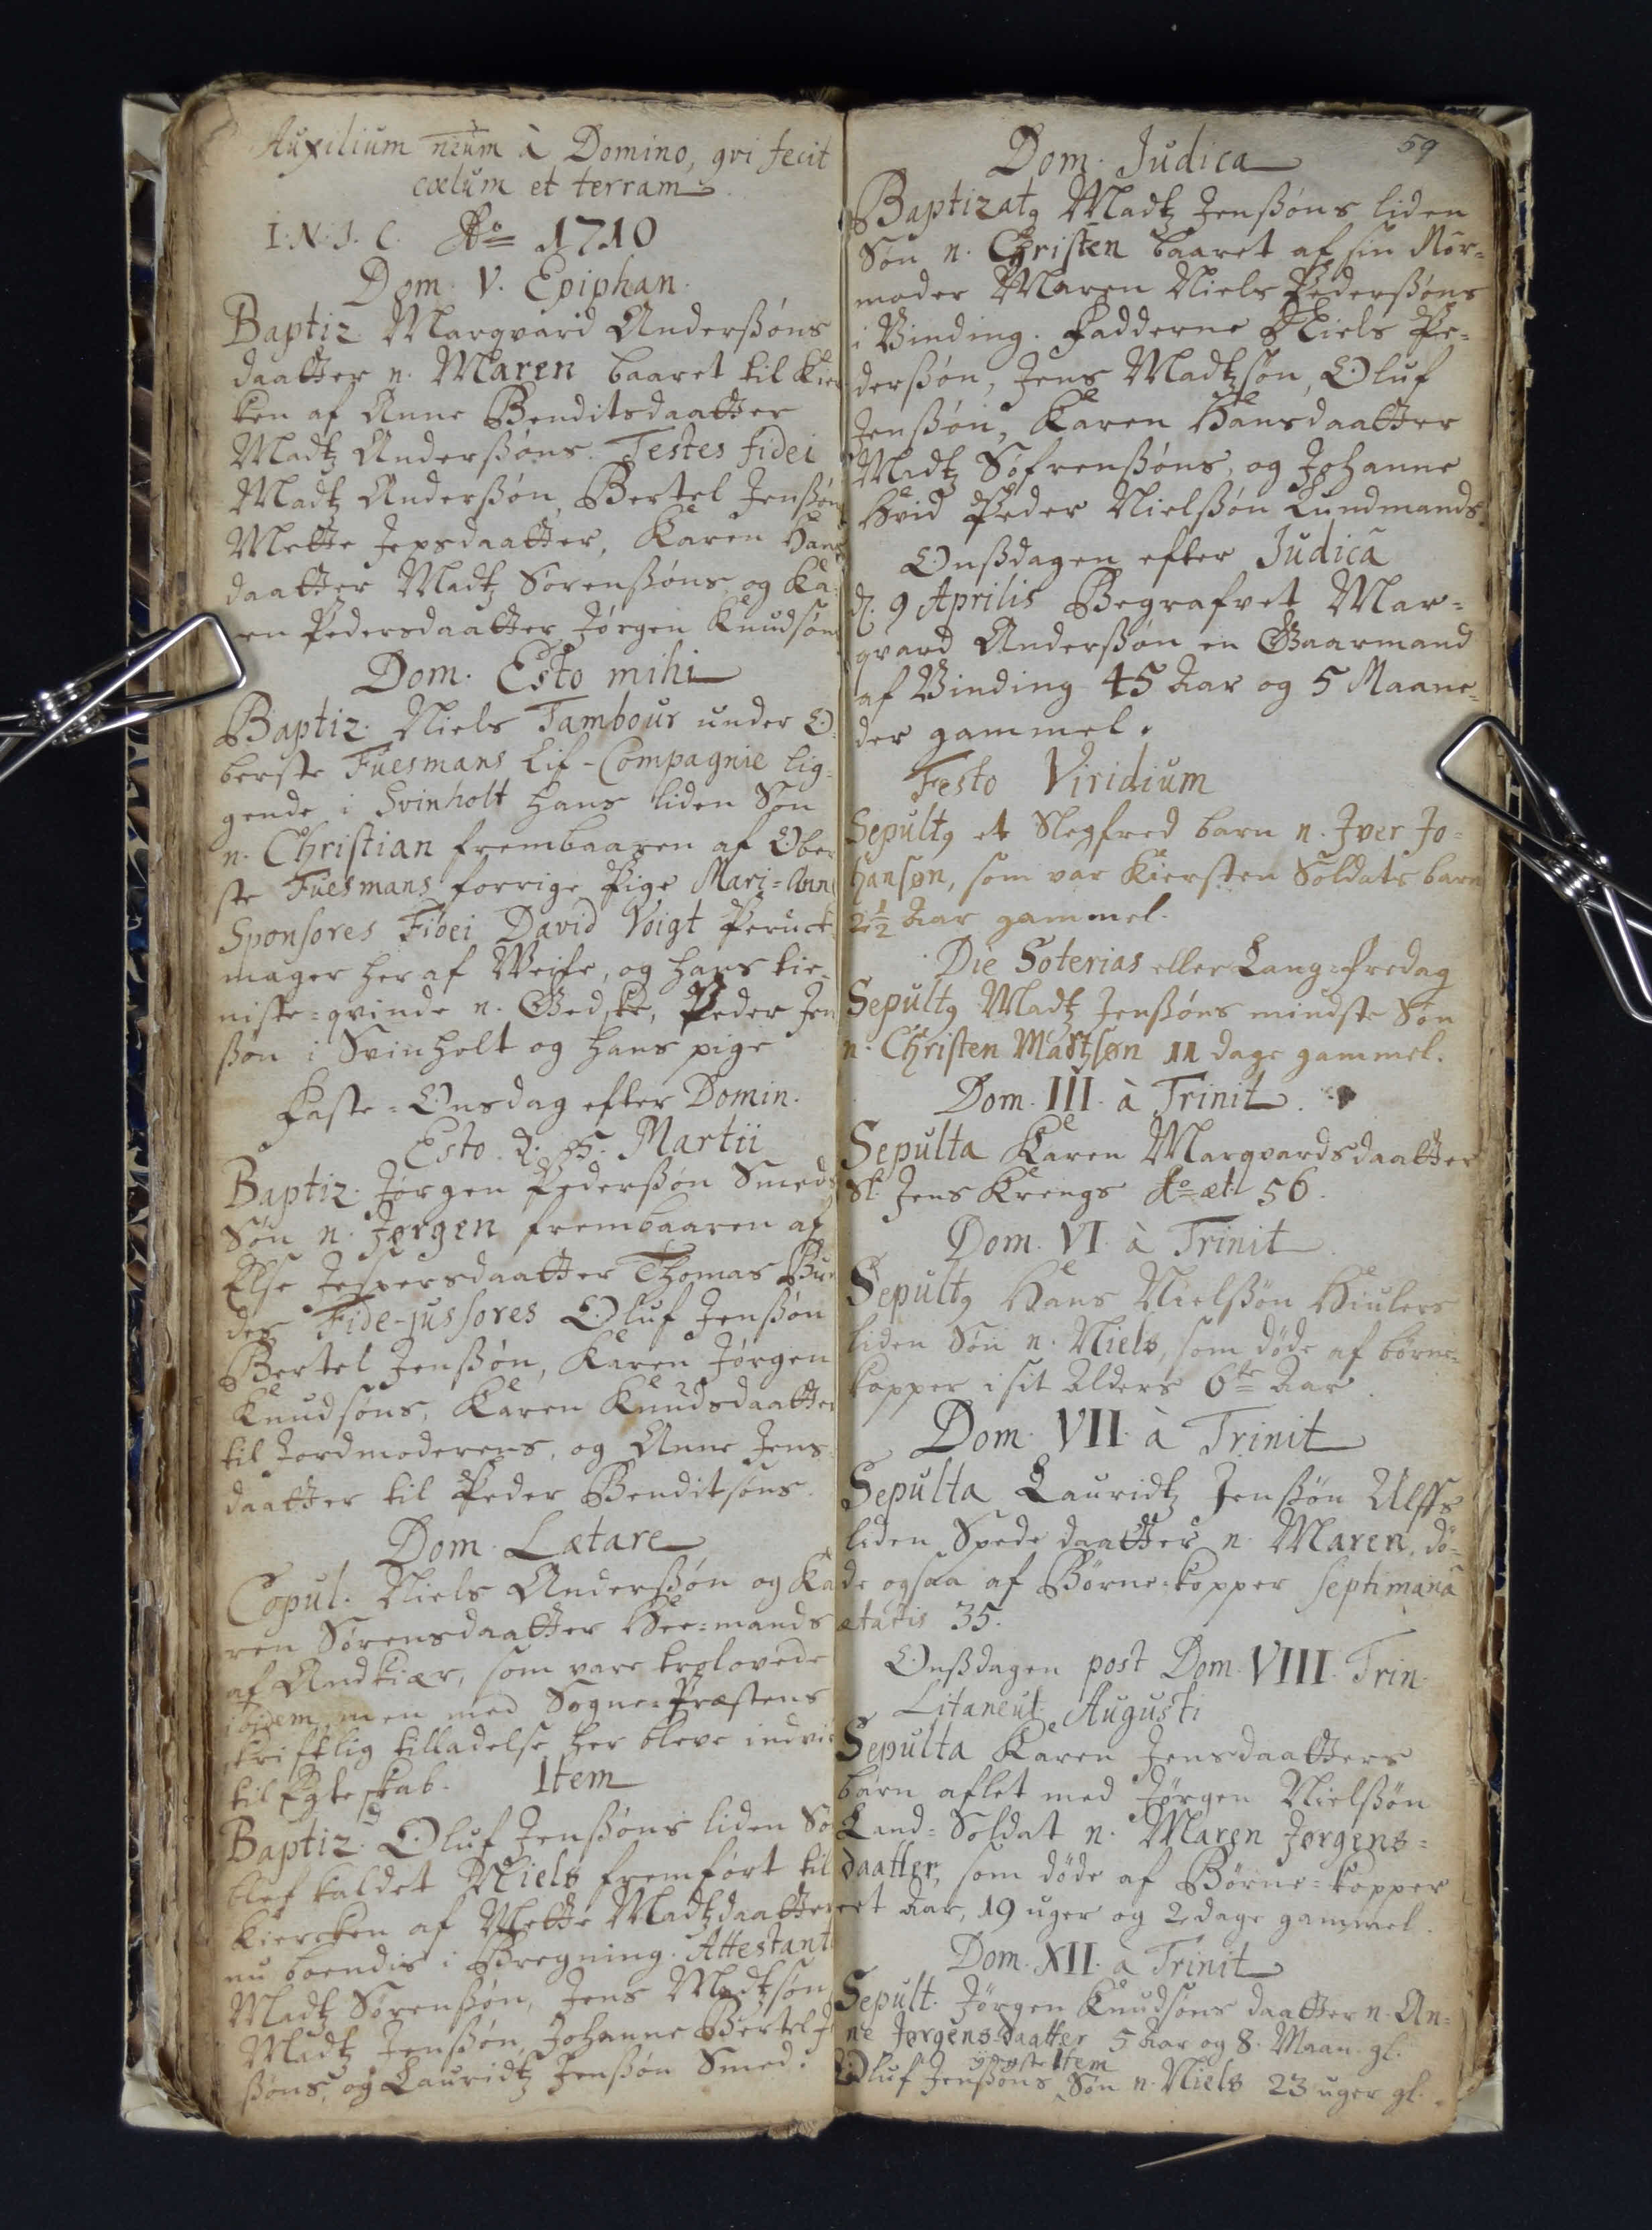Auxilium neum a Domino, qvi fecit
calum et terram
I. N. J. C. Ao 1710
Dom. V. Epiphan
Baptiz Marqvard Anderssøns
daatter n. Maren baaret til Kir¬
ken af Anne Benditsdaatter
Madtz Anderssøns. Testes fidei
Madtz Anderssøn, Bertel Jenssøn.
Mette Jepsdaatter, Karen Hans
daatter Madtz Sørenssøns og Ka¬
en Pedersdaatter Jørgen Knudsøn 
Dom. Esto Mihi
Baptiz. Niels Tambour under O¬
berste Fuesmans Lif-Compagnie lig¬
gende i Svinholt hans liden Søn
n. Christian, frembaaren af
te Fuesmans forrige Pige Mari=Ann
Sponsores Fidei David Voigt Peruck¬
mager her af Weile, og hans tie¬
niste=qvinde n. Gedske, Peder Jen
ssøn i Svinholt og hans pige
Faste = Onsdag efter Domin.
Esto.  d. 5 Martii
Baptiz. Jørgen Pederssøn Smeds
Søn n. Jørgen, frembaaren af
Else Jespersdaatter Thomas Bu
des Fide-jussores Oluf Jenssøn
Bertel Jenssøn, Karen Jørgen
Knudsøns, Karen Knudsdaatter
til Jordmoderens, og Anne Jens¬
daatter til Peder Benditsøns.
Dom. Lætare
Copul. Niels Anderssøn og Ka
ren Sørensdaatter Hee=mands
af Andkiær, som vare trolovede
ibidem, men med Sogne=Præstens
skriftlig tilladelse her bleve
til Egteskab.
Item
Baptiz. Oluf Jenssøns liden Sø
blef kaldet Niels fremført til
Kierken af Mette Madtzdaatterts
nu boendis i Breining. Attestant
Madtz Sørenssøn, Jens Madtzsøn
Madtz Jenssøn, Johanne Bertel J 
ssøns, og Lauridtz Jenssøn Smed.
59
Dom. Judica
Baptizatq Madtz Jenssøns liden
Søn n. Christen baaret af sin Mor¬
moder Maren Niels Pederssøns
i Vinding. Fadderne Niels Pe¬
derssøn, Jens Madtzøn, Oluf
Jenssøn, Karen Hansdaatter
Madts Søfrenssøns, og Johanne
Hvid Peder Nielssøn Lundmands.
Onssdagen efter Judica
d. 9 Aprilis Begrafvet Mar¬
qvard Anderssøn en Gaarmand
af Vinding 45 Aar og 5 Maane¬
der gammel.
Festo Viridium
Sepultq et Slegfred barn n. Iver Jo¬
hansøn, som var Kiersten Soldats barn
2½ Aar gammel.
Die Soterias eller Lang=Fredag
Sepultq Madtz Jenssøns mindste Søn
n. Christen Madtzøn 11 dage gammel.
Dom. III. a Trinit.
Sepulta Karen Marqvardsdaatter
Sl. Jens Krengs Ao æt 56
Dom. VI. a Trinit
Sepultq Hans Nielssøn Hiulers
liden Søn n. Niels, som døde af børne¬
kopper i sit Alders 6te Aar
Dom. VII. a Trinit
Sepulta Lauridtz Jenssøn Ulffs
liden Spæde daatter n. Maren, dø¬
de ogsaa af Børne=Kopper Septimana
ætatis 35
Onssdagen post Dom. VIII. Trin.
Litaneut Augusti
Sepulta Karen Jensdaatters
barn aflet med Jørgen Nielssøn
Land=Soldat n. Maren Jørgens¬
daatter, som døde af Børne = Kopper
eet Aar, 19 uger og 2 dage gammel.
Dom. XII. a Trinit
Sepult. Jørgen Knudsøns daatter n. An¬
Item
yngste
ne Jørgensdaatter 5 Aar og 8 Maaneder gl.
Oluf Jenssøns Søn n. Niels 23 uger gl.